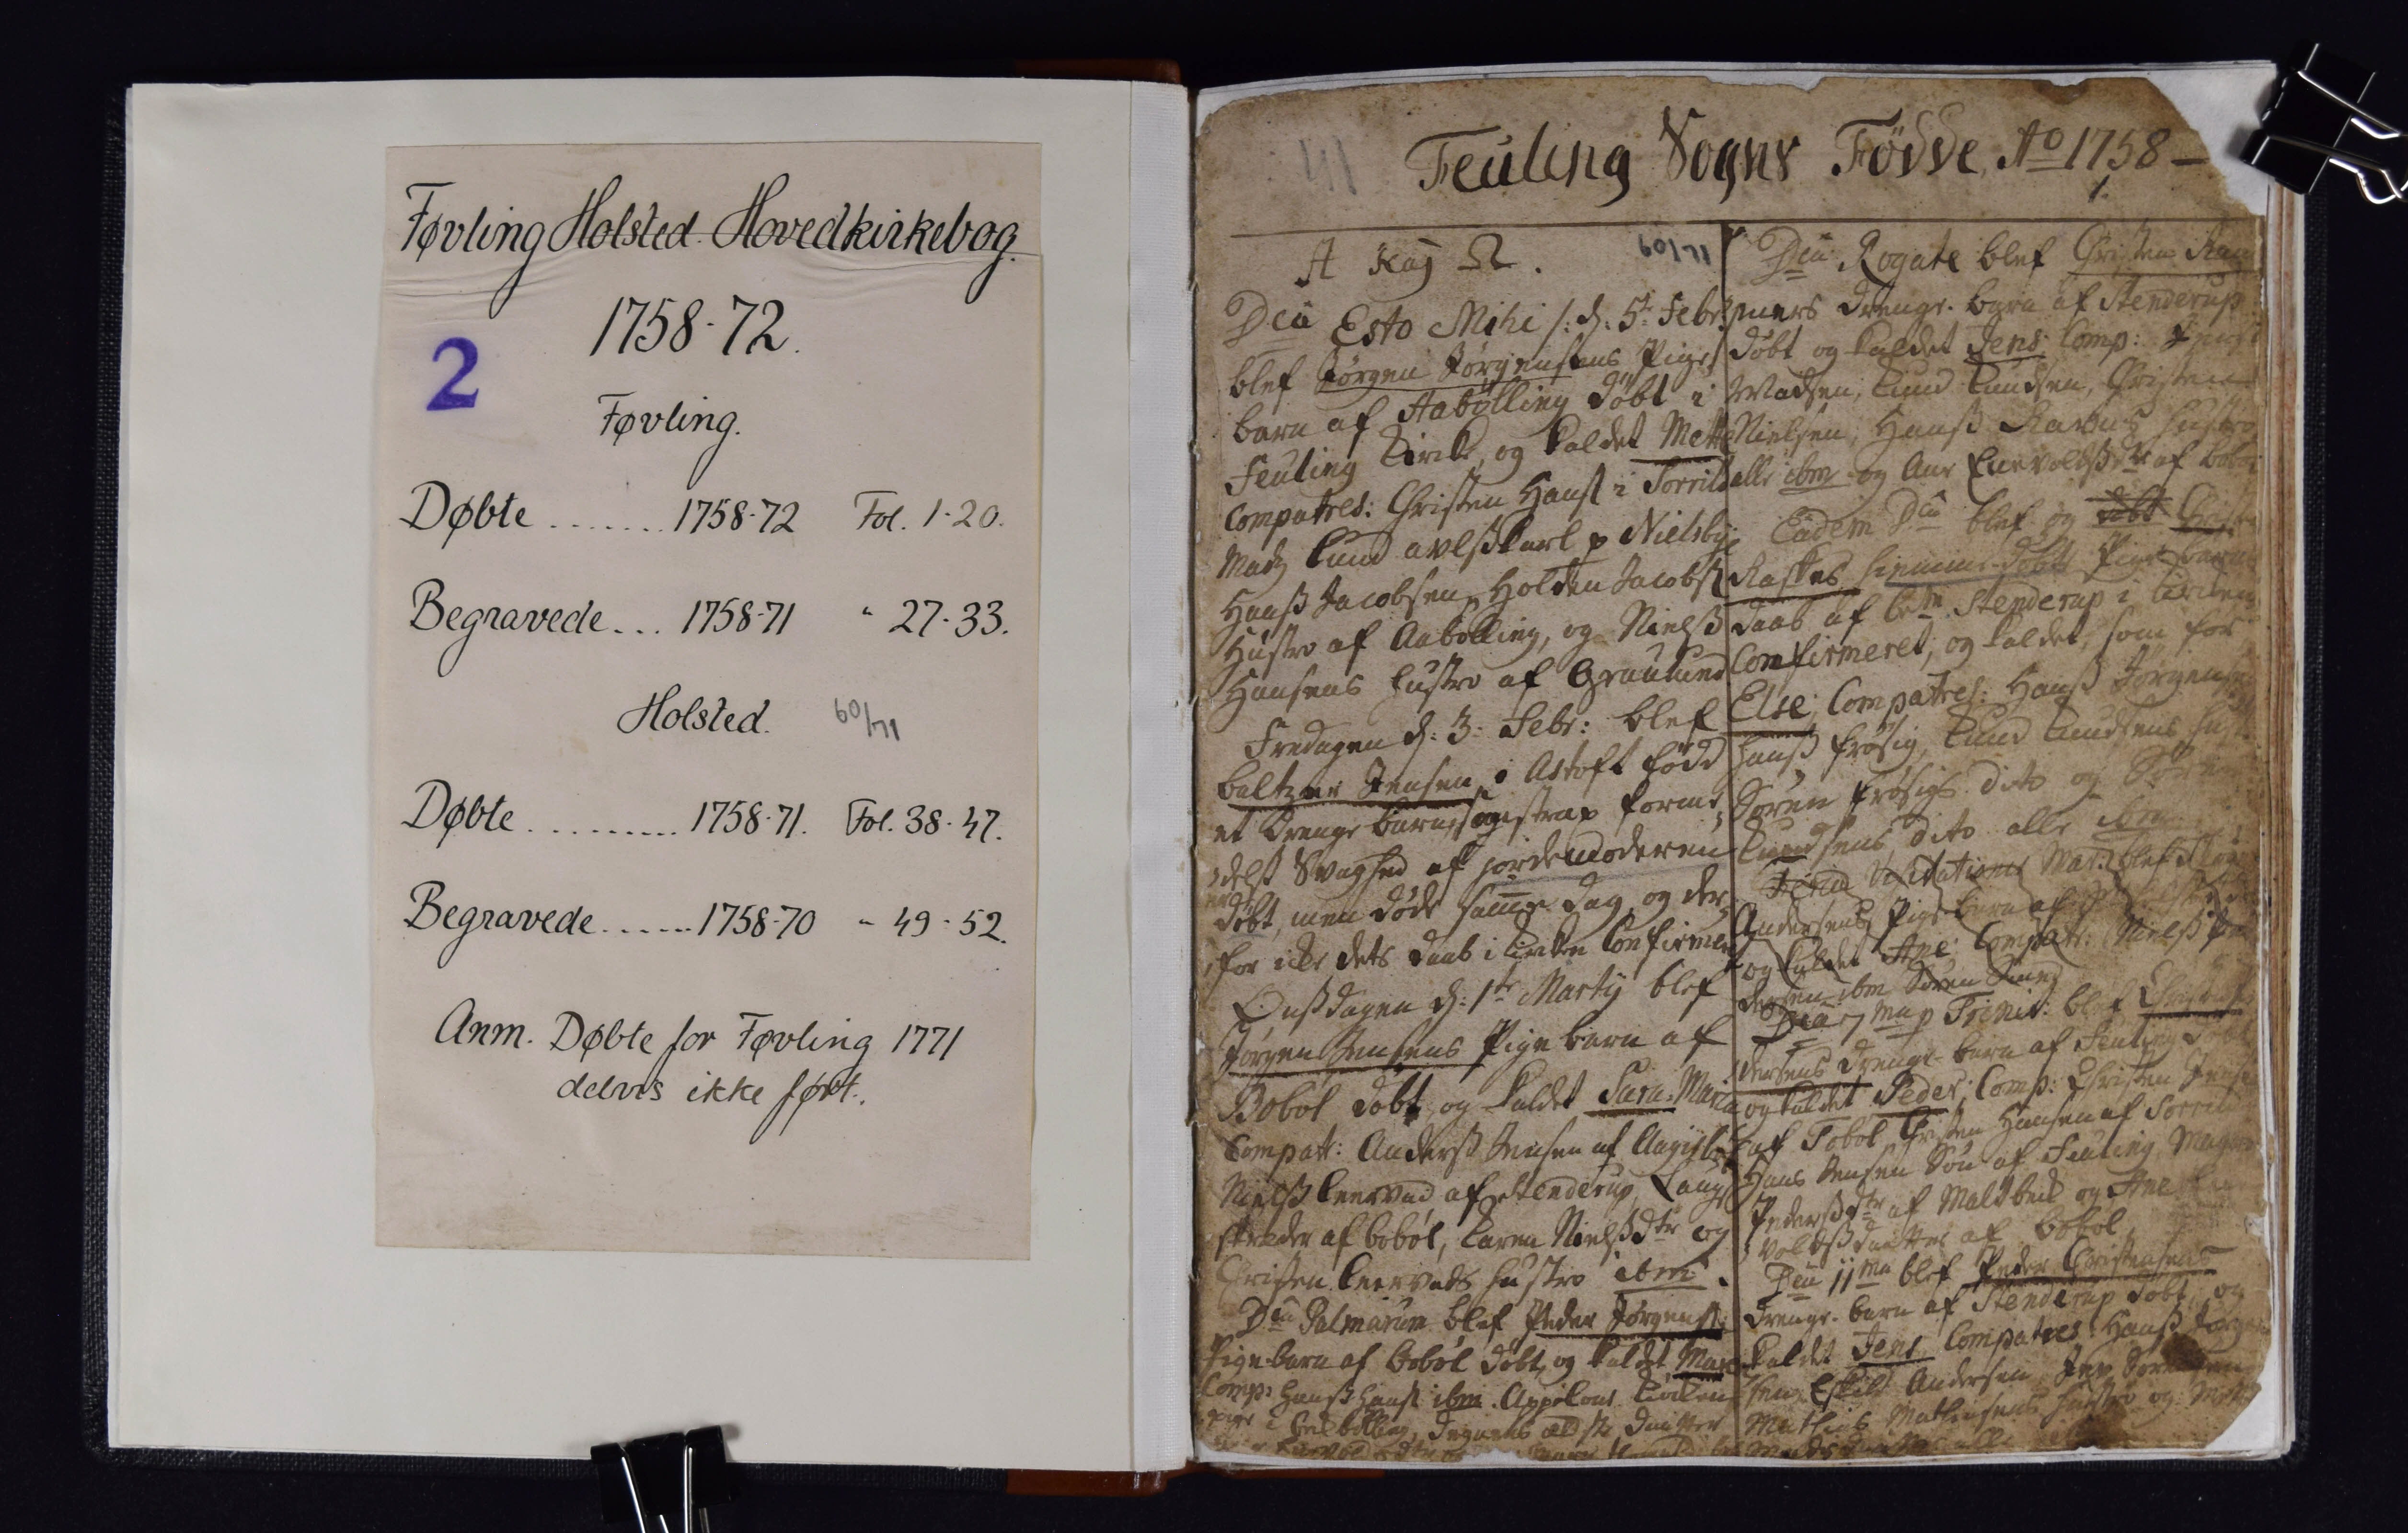Feuling Sogns Fødde Ao 1758 -
60/71
A Kaj Ω
Dca Esto Mihi /:d: 5te Febr:
blef Jørgen Jørgensens Pige
Barn af Aabølling døbt i
Feuling Kirke, og kaldet Mette
Compatres: Christen Hans i Sorrild
Madz Lund avlskarl p Nielsbye
Hans Jacobsen Holden Jacobs
Hustro af Aabølling, og Nielss
Hansens Hustro af Graulund
Fredagen d: 3 Febr: blef
Baltzar Jensen i Astoft, fødd
et Drengebarn strax forme
delst Svaghed af Jordemoderen
døbt, men døde samme dag og der
for icke dets Daab i Kirken Confirmeret
Onsdagen d: 1ste Martij blef
Jørgen Jensens Pigebarn af
Bobøl døbt og kaldet Sara Maria
Compatr: Anders Jensen af Aagisbøl
Nielss Leervad af Stenderup, Lauge
Skreder af bobøl, Karen Nielsdtr og
Christen Leervads hustro ibm.
Dca Palmarum blef Peder Jørgens
Pigebarn af Bobøl døbt og kaldet Mare
Comp: Hanss Hans ibm. Appelone Kiøken
pige i bekbølling, Degnens ælste daatter
## Enevoldsdtr
## ##
Dca Rogate blef Christen Ram
mers Drenge Barn af Stenderup
døbt og kaldet Jens Comp: Jens
Madsen, Knud Knudsen, Christen
Nielsen Hanss Rasmus Hustro
alle ibm og Ane Enevoldsdtr af Bobøl
Eodem Dca blef og døbt Christ##
Raskes hiemmedøbt Pige Barns
Daab af bem Stenderup i Kirken
Confirmeret; og kaldet som før
Else, Compatres: Hans Jørgens
Hans Frøsig, Knud Knudsens Hustro
Søren Frøsigs dito og Søren
Kundsens dito alle ibm
Feria Visitationis Mar: blef ##
Andersens Pige Barn af ## ##
og kaldet Ane Compatr: Nielss Pe
dersen ibm: Søren S##
Dca 7ma p Trinit: blef Christen Pe
derses Drenge Barn af Feuling døbt
og kaldet Peder; Comp: Christen Jensen
af Tobøl, Christen Hansen af Sorrild
Hans Jensen Søn af Feuling Mag##
Pederssdtr af Maltbeik og Ane Ene
voldssdatter af Bobøl,
Dca 11ma blef Peder Christensens
Drenge Barn af Stenderup døbt og
kaldet Jens Compatres Hanss Jørgen
sens Eskild Andersen, Jens Sørensen
Mathias Mathiasens hustro og M##
Mads Datter alle ##
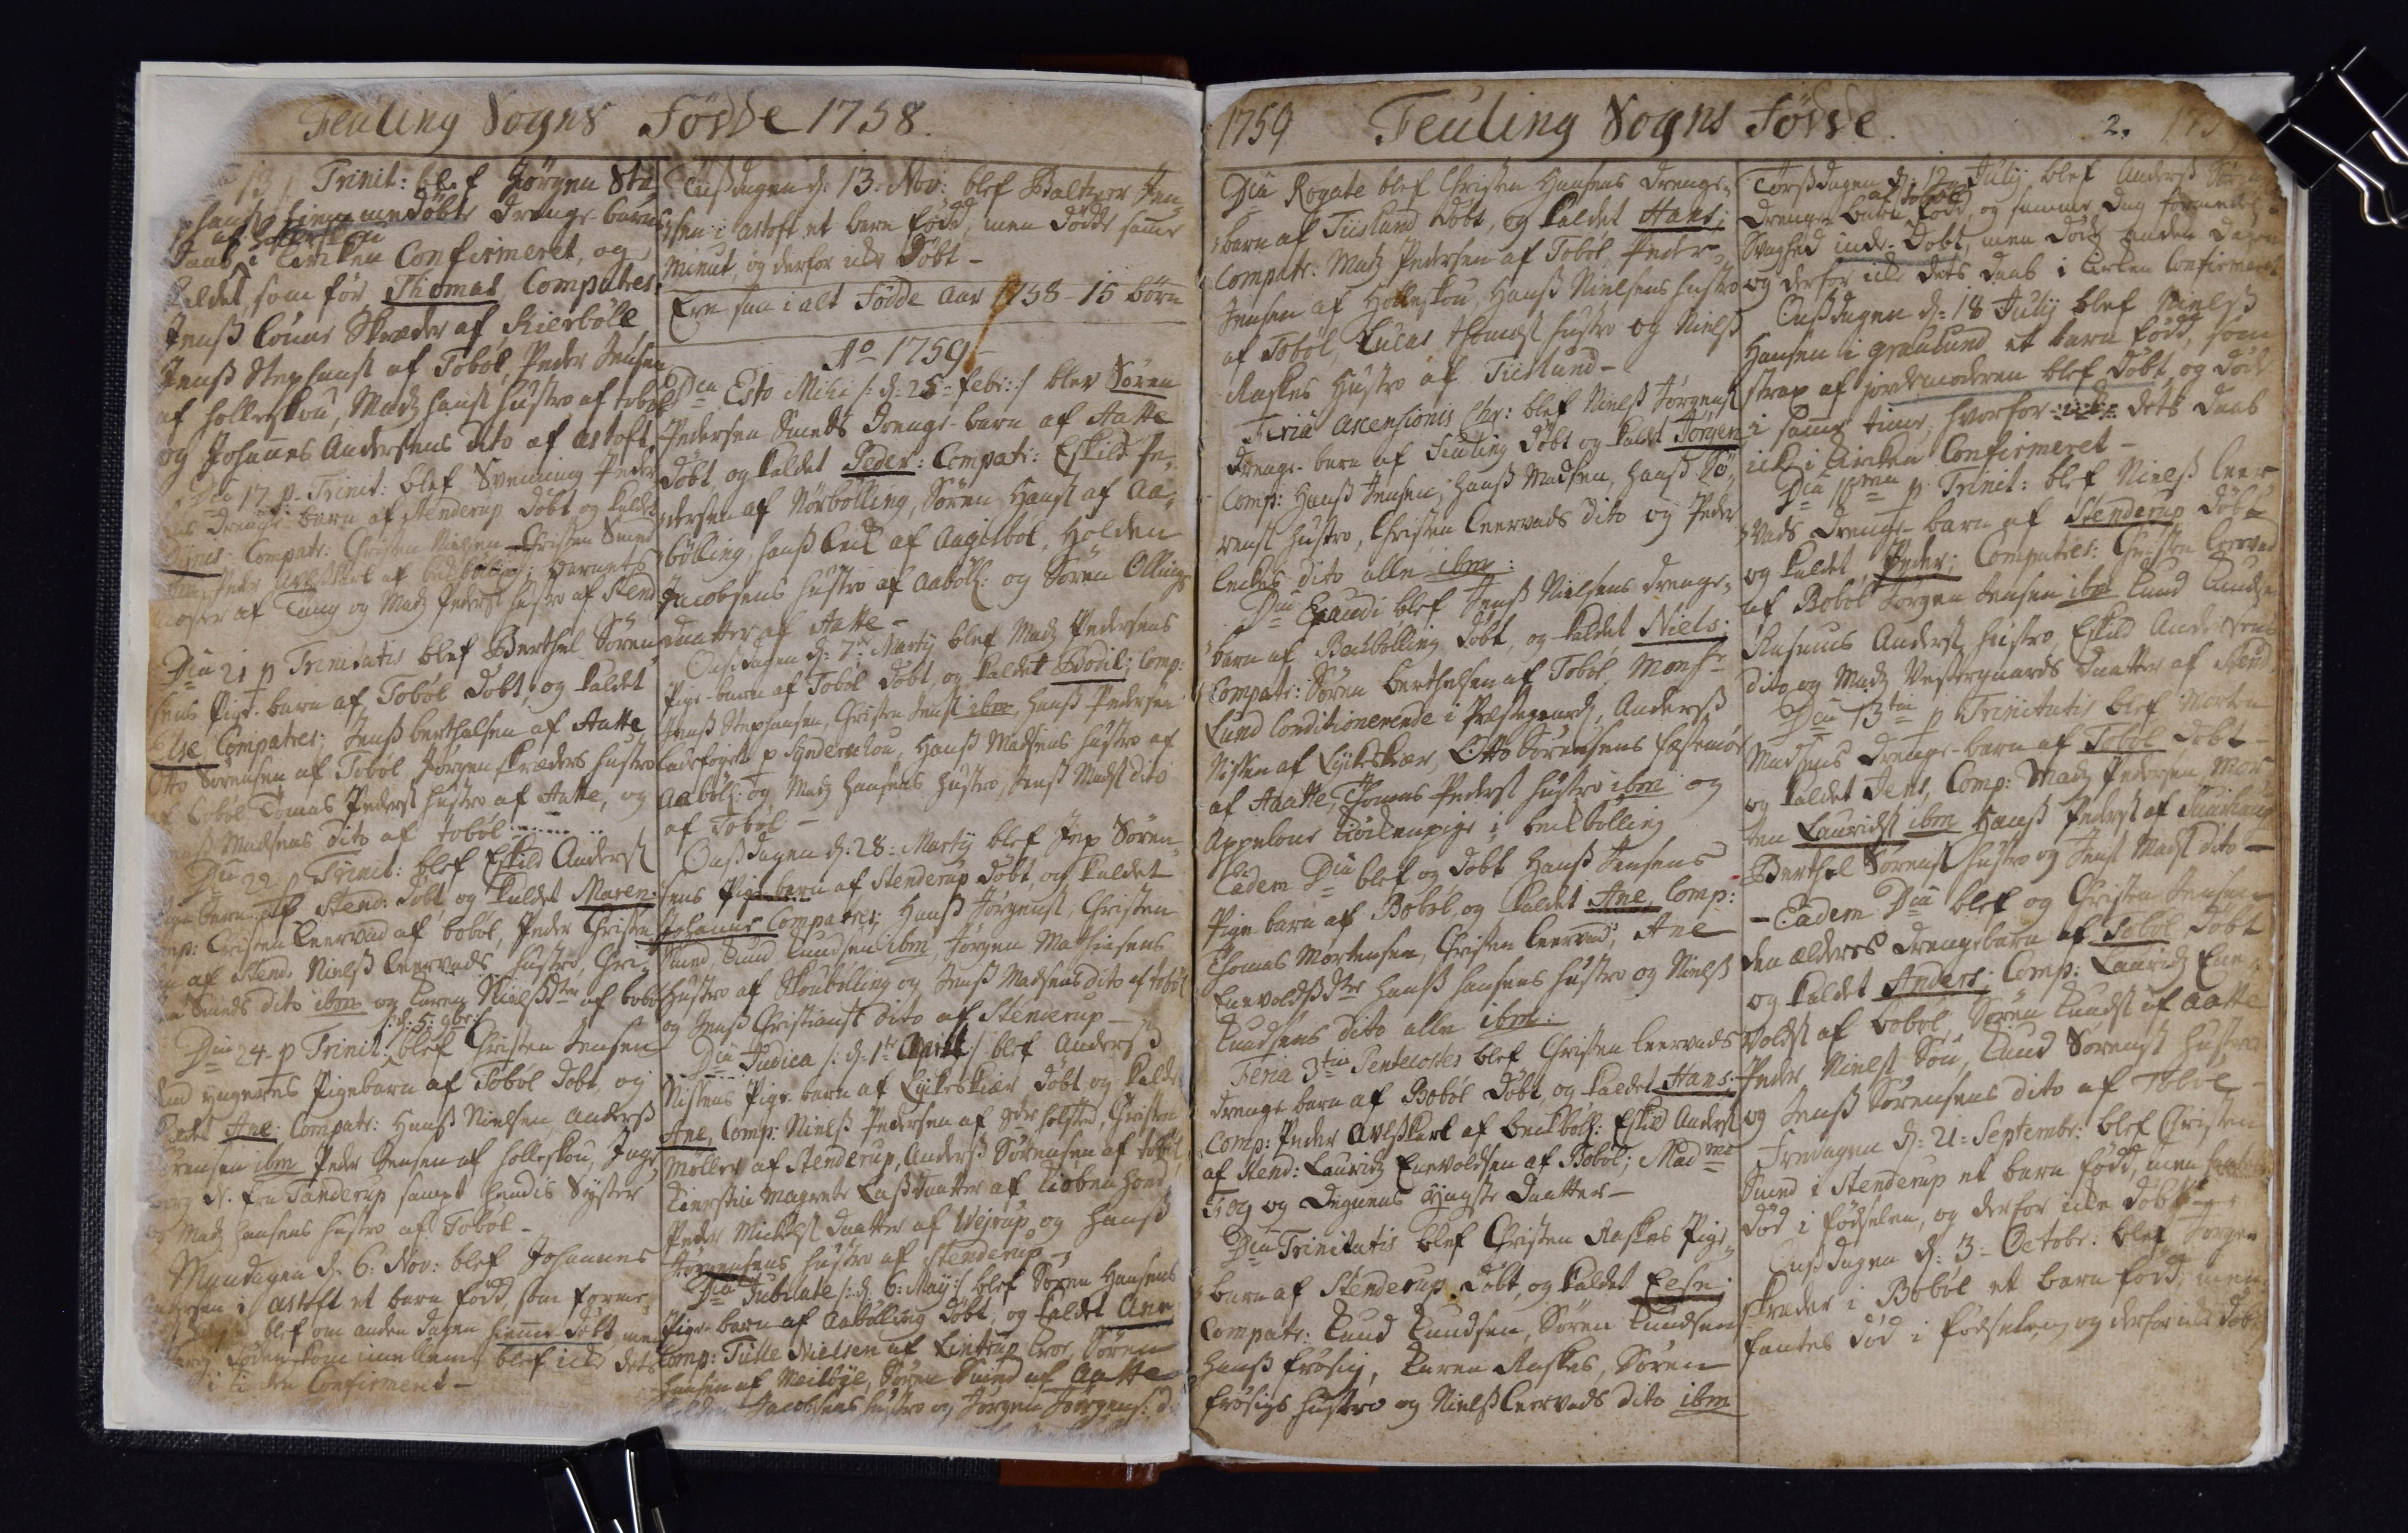Feuling Sogns Fødde 1758.
Dca 13 p Trinit: bleff Jørgen Ste¬
phans hiemmedøbt Drenge Barns
af Holleskou
Daab i Kirken Confirmeret, og
kaldet som før Thomas Compatres
Jens Loune Skræder af Kierbøle.
Jens Stephans af Tobøl Peder Jensen
af Holleskou Madz Hans Hustro af Tobøl
og Johannes Andersens dito af Astoft.
Dca 17 p. Trinit: blef Svenning Peder
sens Drenge Barn af Stenderup døbt og kaldet
Dynis Compatr: Christen Nielsen, Christen Smed
ibm Peder Avlskarl af Bekbøling; Barnet
Moster af Tang og Madz Peders Hustro af Stend
D 21 p Trinitatis blef Berthel Søren
sens Pige Barn af Tobøl døbt og kaldet
Else Compatres: Jens Berthelsen af Aatte
Otto Sørensen af Tobøl Jørgen Skræders Hustro
af Tobøl, Tomas Peders Hustro af Aatte og
##enss Madsens dito af Tobøl . . . . . . .
Dca 22. p Trinit: bleff Eskild Anders
##ige Barn af Stend: døbt og kaldet Maren.
##mp: Christen Leervad af Bobøl, Peder Christen¬
## af Stend: Niels Leervad Hustro Chri¬
sten Smeds dito ibm og Karen Nielssdtr af bobø 
##: d: 5: 9br:
Dca 24. p Trinit: bleff Christen Jensen
den yngeres Pigebarn af Tobøl døbt og
kaldet Ane Compatr: Hans Nielsen Anders
Sørensøn ibm Peder Jensen af Holleskou, Inge¬
borg ##. fra Tanderup sampt hendis Syster
og Madz Hansens Hustro af Tobøl.
Mandagen d: 6: Nov: blef Johannes
##dersen i Astoft et barn fødd som forme¬
## ## blef om anden dagen hiemmedøbt med
## ## kom imellem blef icke dets
## Confirmeret-
Tisdagen d: 13. Nov: blef Baltzer Jen
sen i astoft et barn fødd, men dødde samme
minit, og derfor icke Døbt -
Ere saa i alt Fødde Aar 1758 15 Børn
Ao 1759
Dca Esto Mihi: /: d: 25 Febr: :/ blev Søren
Pedersen Smeds Drenge-barn af Aatte
døbt og kaldet Peder: Compatr: Eskild Pe¬
dersen af Nørbølling, Søren Hans af Aa¬
bølling, hans ##k af Aagi##bøl, Holden
Jacobsens Hustro af Aabøll: og Søren Ollings
Daatter af Aatte -
Onssdagen d: 7de Martij blev Madz Pedersens
Pige-barn af Tobøl døbt, og kaldet Bodil, Comp:
Hans Stephansen, Christen Jens ibm, Hans Pedersen
Ladefoget p Synderschou, Hanss Madsens Hustro af
Aabøll: og Madz Hansens Hustro, Jens Mads dito
af Tobøl
Onssdagen d. 28: Martij blef Jep Søren
sens Pige-barn af Stenderup døbt og kaldet
Johanne Compatres: Hans Jørgens, Christen
Smed, Knud Knudsen ibm, Jørgen Mathiasens
Hustro af Skoubølling og Jens Madsens dito af Tobøl
og Jens Christians dito af Stenderup
Dca Judica /: d: 1te April bl :/ blef Anderss
Nissens Pige-barn af Kykeskiær døbt og kaldet
Ane Comp: Nielss Pedersen af Sdr holsted, Christen
Møller af Stenderup, Anders Sørensen af Tobøl
Kierstine Margrete Lass Daatter af Kiøben Hoed
Peder Mickels Daatter af Vejrup og Hans
Jørgensens Hustro af Stenderup
Dca Jubilate /: d: 6: Maij :/ blef Søren Hansens
Pige-barn af Aabølling døbt og kaldet Ane
Comp: Tulle Nielsen af Lintrup Kroe. Søren
Hansen af Meilbye, Søren Smid af Aatte
Holden Jacobsens Hustro og Jørgen Jørgens: d##
1759
Feuling Sogns Fødde
Dca Rogate blef Christen Hansens Drenge¬
barn af Tiislund døbt, og kaldet Hans;
Compatr: Matz Pedersen af Tobøl Peder
Jensen af Holleskou, Hans Nielsens Hustro
af Tobøl, Lucas Thomæs Hustro og Niels
Raskes Hustro af Tiislund-
Feria Ascensionis Chr: blef Niels Jørgens
Drenge-barn af Feuling døbt og kaldet Jørgen
Caop: Hanss Jensen, Hanss Madsen, Hans Sø¬
rens Hustro, Christen Leervads dito og Peder 
Leckes dito alle ibm
Dca Exaudi blef Hans Nielsens denge¬
barn af Bechbølling døbt og kaldet Niels
Compatr: Søren Berthelsen af Tobøl Monsr
Lund Conditionerende i Præstegaard, Anders
Nissen af Lykeskiær, Otte Sørensens Fæstemø
af Aaatte, Tomas Peders Hustro ibm og
Appellone Kiøkenpige i beckbølling
Eodem Dca blef og døbt Hanss Jensens
Pige barn af Bobøl, og kaldet Ane Comp:
Tomas Mortensen, Christen Leervad, Ane
Envoldssdtr Hanss Hansens Hustro og Niels
Knudsens dito alle ibm:
Feria 3tia Pentecostes blef Christen Leervads
drenge barn af Bobøl døbt og kaldet Hans.
Comp: Peder Avlskarl af Beckbøll: Eskild Anders
af Stend: Lauridz Enevoldsen af Bobøl; Madme
Fog og Degnens yngste Daatter-
Dca Trinitatis blef Christen Raskes Pige¬
Barn af Stenderup døbt, og kaldet Else
Compatr: Knud Knudsen, Søren Knudsen
Hans Frøsig, Karen Raskes, Søren
Frøsigs Hustro og Nielss Leervads dito ibm.
2.
17##
Torsdagen d: 12 Julij blef Anders Sørensens
af Tobøl
Drenge= Barn ^ fødd og samme Dag formedelst
Svaghed ind- Døbt, men død anden Dagen
og derfor icke dets Daab i Kirken Confirmeret
Onsdagen d: 18 Julij blef Nielss
Hansen i Graulund et barn fødd, som
strax af jordemoderen blef døbt og døde
samme timer, hvorfor icke dets Daab
icke i Kirken Confirmeret-
Dca 10ma p Trinit: blef Niels Lars¬
=sens Drenge- barn af Stenderup døbt
og kaldet Peder; Compatres: Christen Larsens
af Bobøl Jørgen Jensen ibd Knud Knudsen
Rasmus Anders Hustro Eskild Andersens
dito og Madz Vestergaards Daatter af Stend
Dca 13tia p Trinitatis blef Morten
Madsens Drenge-barn af Tobøl døbt
og kaldet Jens Comp: Madz Pedersen Mor
men Laurids ibm Hans Peders af Suurhaug
Berthel Sørens Hustro og Jens Mads dito-
Eadem Dca blef og Christen Jensen
denher ældres Drengebarn af Tobøl døbt
og kaldet Anders Comp: Lauridz Ene
volds af bobøl, Søren Knuds af Aatte
Peder Niels Søn, CKnud Sørens Hustro
og Jens Sørrensens dito af Tobøl
Fredagen d: 21: Septembr: blef Christen
Smed i Stenderup et barn fødd, men fantes
død i Fødselen, og derfor ikke døbt ##
Onssdagen d: 3 Octobr. blef Jørgen
Skræder i Bobøl et barn fødd, men
fantes død i Fødselen, og derfor icke døbt